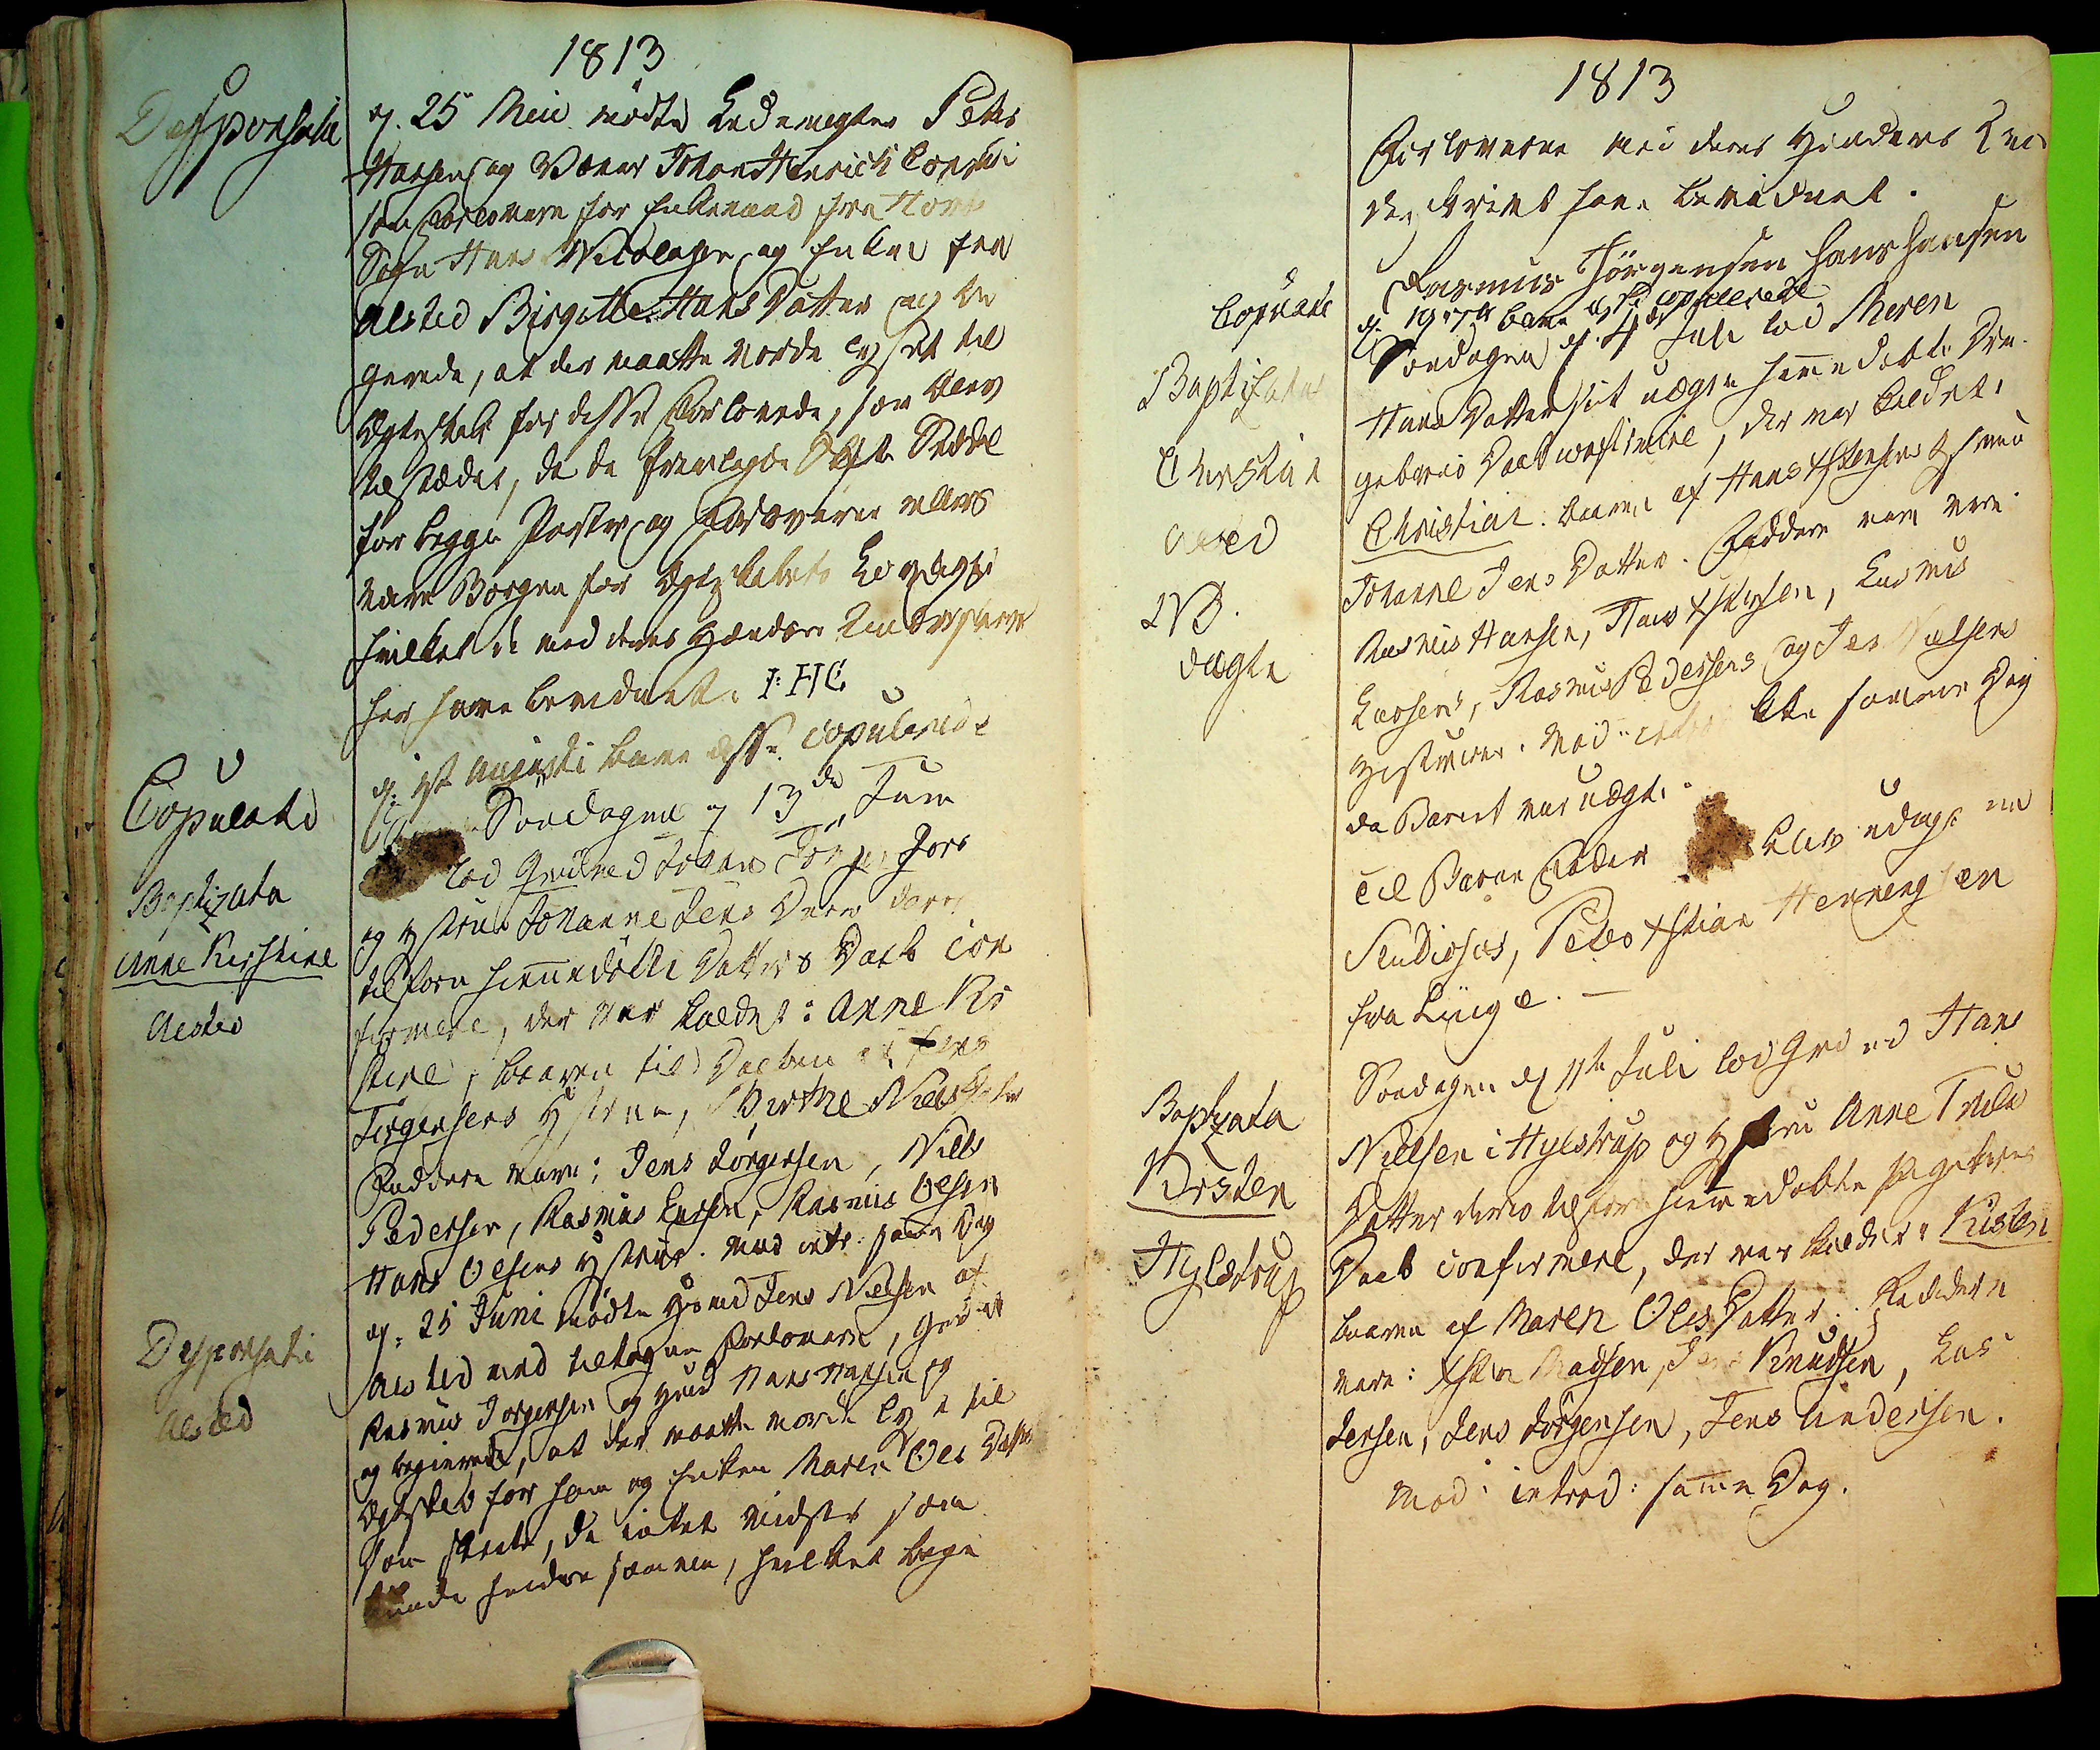Desponsati
Copulati
Baptizata
Anne Kirstine
Alsted
Desponsati
Alsted
1813
d 25 Mai mødte Lede## Peter
Hansen og Væver Johan Henrich Co##
som Forlovere for Enkemand fra Ho##
Sogn Hans Nicolasen og Enken fra
Alsted Birgitte Hans Datter og be
gerede, at der maatte vorde lyst til
Ægteskab for disse Frlovede, som blev
tilstædet, da de fremlagde Skilfte Seddel
for begge Parter og Forloverne ##
vare Borgen for Ægteskabet Lovlighed
hvilket de med deres Hænders Underskrivt
her have bevidne. I H C
d: 1ste Augusti bleve disse copulerede
Søndagen d 13de  Jun
lod Grdmd Johans Søn Jørgensen##
og Hstrue Johanne Jens Dat deres
tilforn hiemmedøbte Datters Daab con
firmere, der var kaldet: Anne Kir
stine, baaren til Daaben af Jens
Jørgensens Hstrue, Birthe Nielsdatter
Faddere vare: Jens Jørgensen, Niels
Pedersen, Rasmus Larsen, Rasmus Olsen
Hans Olsens Hstrue. Mod: intr: samme Dag
d: 25 Juni mødte Hsmd Jens Nielsen af
Alsted med tiltagne Forlevere, Grdmd
Rasmus Jørgensen og Hmd Hans Hansen og
og begierede, at det Maatte vorde Lyst til
Ægteskab for ham og Enken Maren Ols Datter
## ##, da intet vidstes som
kunde hindre ##, hvilket bege
Copulati
Baptizatus
Christian
Alsted
NB.
uægtedage
Baptizata
Kirsten
Hylstrup
1813
Forloverne ved deres Hænders Un
derskrivt have bevidnet.
Rasmus Jørgenssen Hans Hansen
d: 19## 7br p: bleve disse copulerede
Søndagen d 4de Juli lod Maren
Hans Datter sit uægte hemmedøbte  Dre
gebarns Daab confirmere, der var kaldet:
Christian. baaren af Hans Hansens Hstru
Johanne Jens Datter. Faddere vare
Rasmus Hansen, Hans Xstensen, ##mus
Larsen, Rasmus Pedersens og Je## Nielsens
Hstruer. Mod introd ikke samme Dag
da Barnet var uægte.
Til Barne Fader blev udlagt en
Studiosus, Peter Xstian Henningsen
fra Liuge.
Søndagen d 11te Juli lod Grdmd Hans
Nielsen i Hylstrup og Hstru Anne Truels
Datter deres tilforn hiemmedøbte Pigebarns
Daab confirmere, der var kaldet: Kirsten
baaren af Maren Oles Datter. Faddere
vare: Xsten Madsen, Jens Knudsen, Las
Jensen, Jens Jørgensen, Jens Andersen
Mod introd: samme Dag.
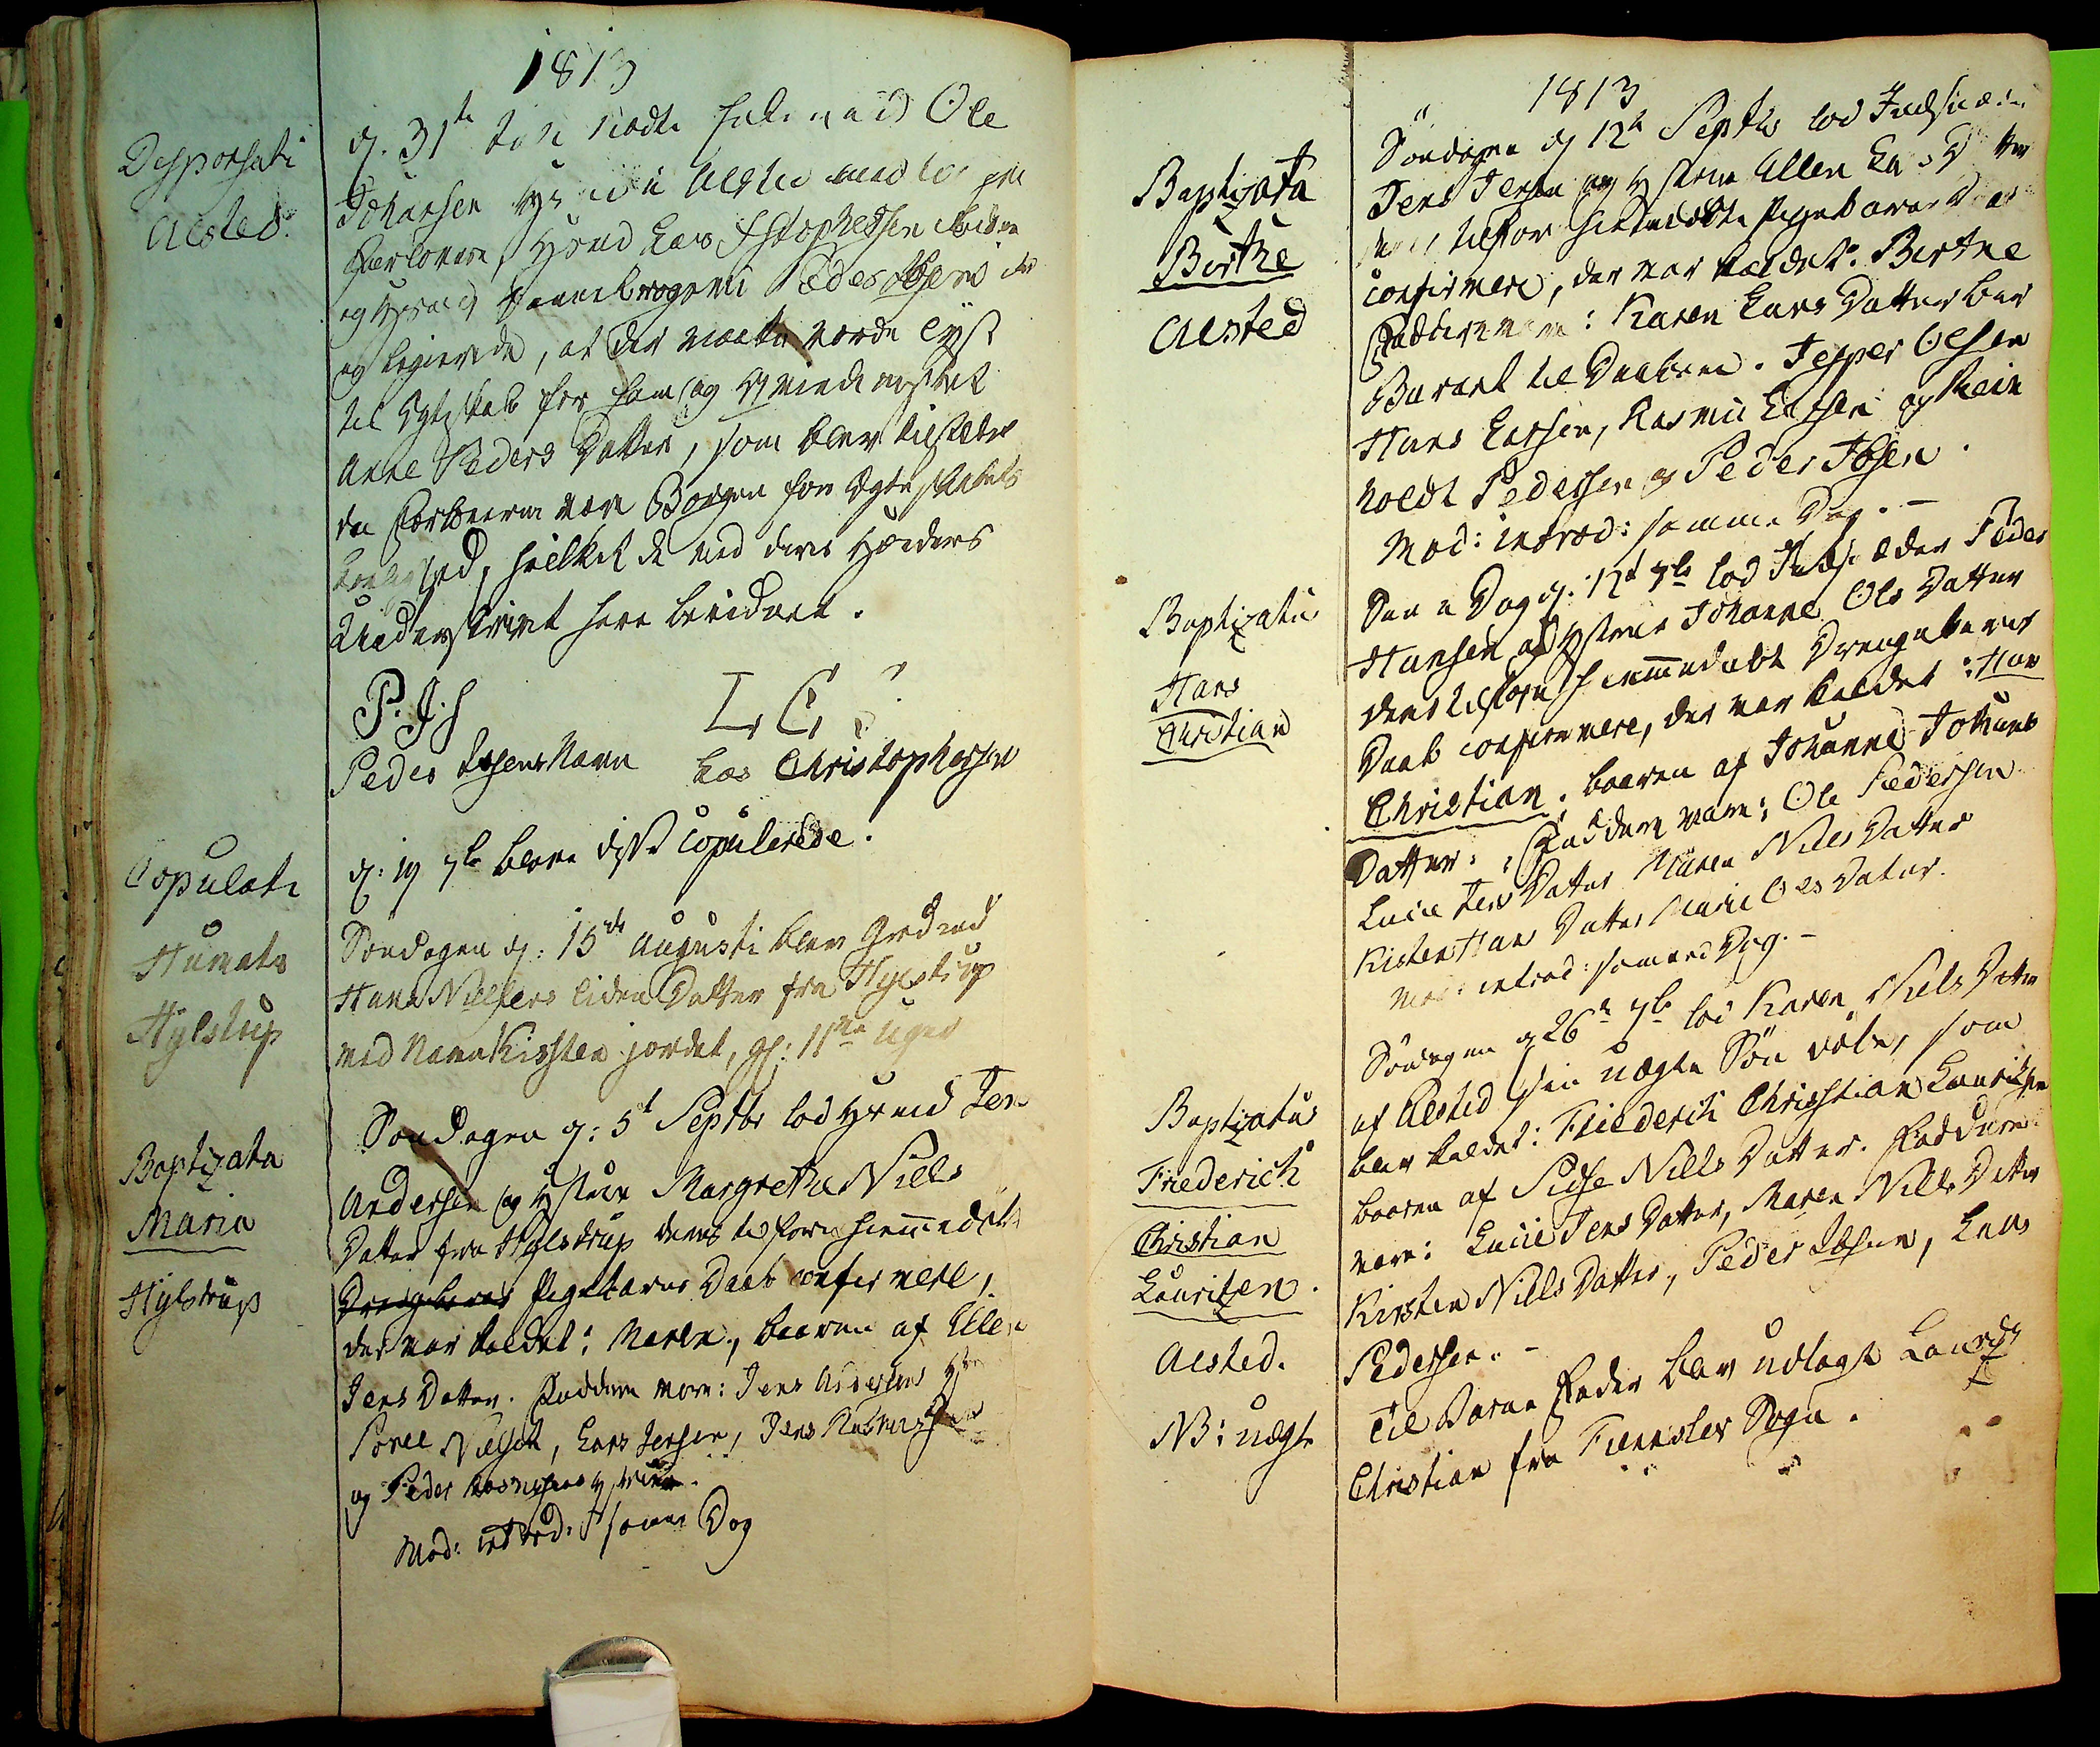Desponsati
Alsted.
Copulati
Humata
Hylstrup
Baptizata
Maria
Hylstrup
1813
d. 31 Juli mødte Enkemand Ole
Johansen Hsmdi i Alsted med tikltagne
Forlevdere, Hsmd Læs Xstophersen ibidem
og Hsmd Dannebrogsmd Peder Ibsens
og begierede, at der maatte vorde lyst
til Ægteskab for ham og
Anne Peders Datter, som blev tilstædet
da Forloverne var Borgen for Ægteskabets
Loivlighed, hvilket de med deres Hænders
Underskrivt have bevidnet.
P. J. S
Peder ##sen Navn
L C S
Las Christophersen
d: 19 7br bleve disse copulerede
Søndagen d: 15de Augusti blev Grdmd
Hans Nielsens liden Datter fra Hylstrup
ved Navn Kirsten jordet, gl: 11ma Uger
Søndagen d: 5te  Septbr lod Hsmd Jens
Andersen og Hstrue Margrethe Niels
Datter fra Hylstrup deres tilforn hiemmedøbte
Drengebarns Pigebarns Daab confirmere,
det var kaldet: Maren, baaren af Ellen
Jens Datter. Faddere vare: Jens Andersens Hstr
Povel Nielsen, Lars Jensen, Jens Rasmusens
og Peder Rasmusens Hstruer.
Mod: introd Samme Dag
Baptizata
Birthe
Alsted.
Baptisatus
Hans
Christian
Baptizatus
Frederich
Christian
Lauritzen
Alsted.
NB: uægte
1813
Søndagen d 12te Septbr lod Indsidder
Jens Jensen og Hstrue Ellen Lars Datter
deres tilforn hiemmdøbte Pigebarns Daab
confirmere, der var kaldet: Birthe
Fadderne vare: Karen Lars Datter bar
Barnet til Daaben. Jesper Olsen
Hans Larsen, Rasmus Larsen og Rein
holdt Pedersen og Peder Ibsen
Mod: introd: samme Dag
Samme Dag d: 12te 7br lod Indsidder Peder
Hansen og Hstrue Johanne Ols Datter
deres tilforn hiemmedøbte Drengebarns
Daab confirmere, der var kaldet: Hans
Christian; baaren af Johanne Johans
Datter. Fadderne vare: Ole Pedersen
Lucie Jens Datter Maren Niels Datter 
Kisten Hans Datter Marie Ols Datter.
Mod: introd: samme Dag.
Søndagen d 26de 7br lod Karen Niels Datter
af Alsted sin uægte Søn døbe, som
bvlev kaldet Friederich Christian Lauritsen
baaren af Sidse Niels Datter. Fadderne
vare: Lucie Jens Datter, Maren Niels Datter
Kirsten Niels Datter, Peder Ibsen, Lars
Pedersen
sTil Barne Fader blev udlagt Lauri##
Christian fra Fienneslev Sogn.